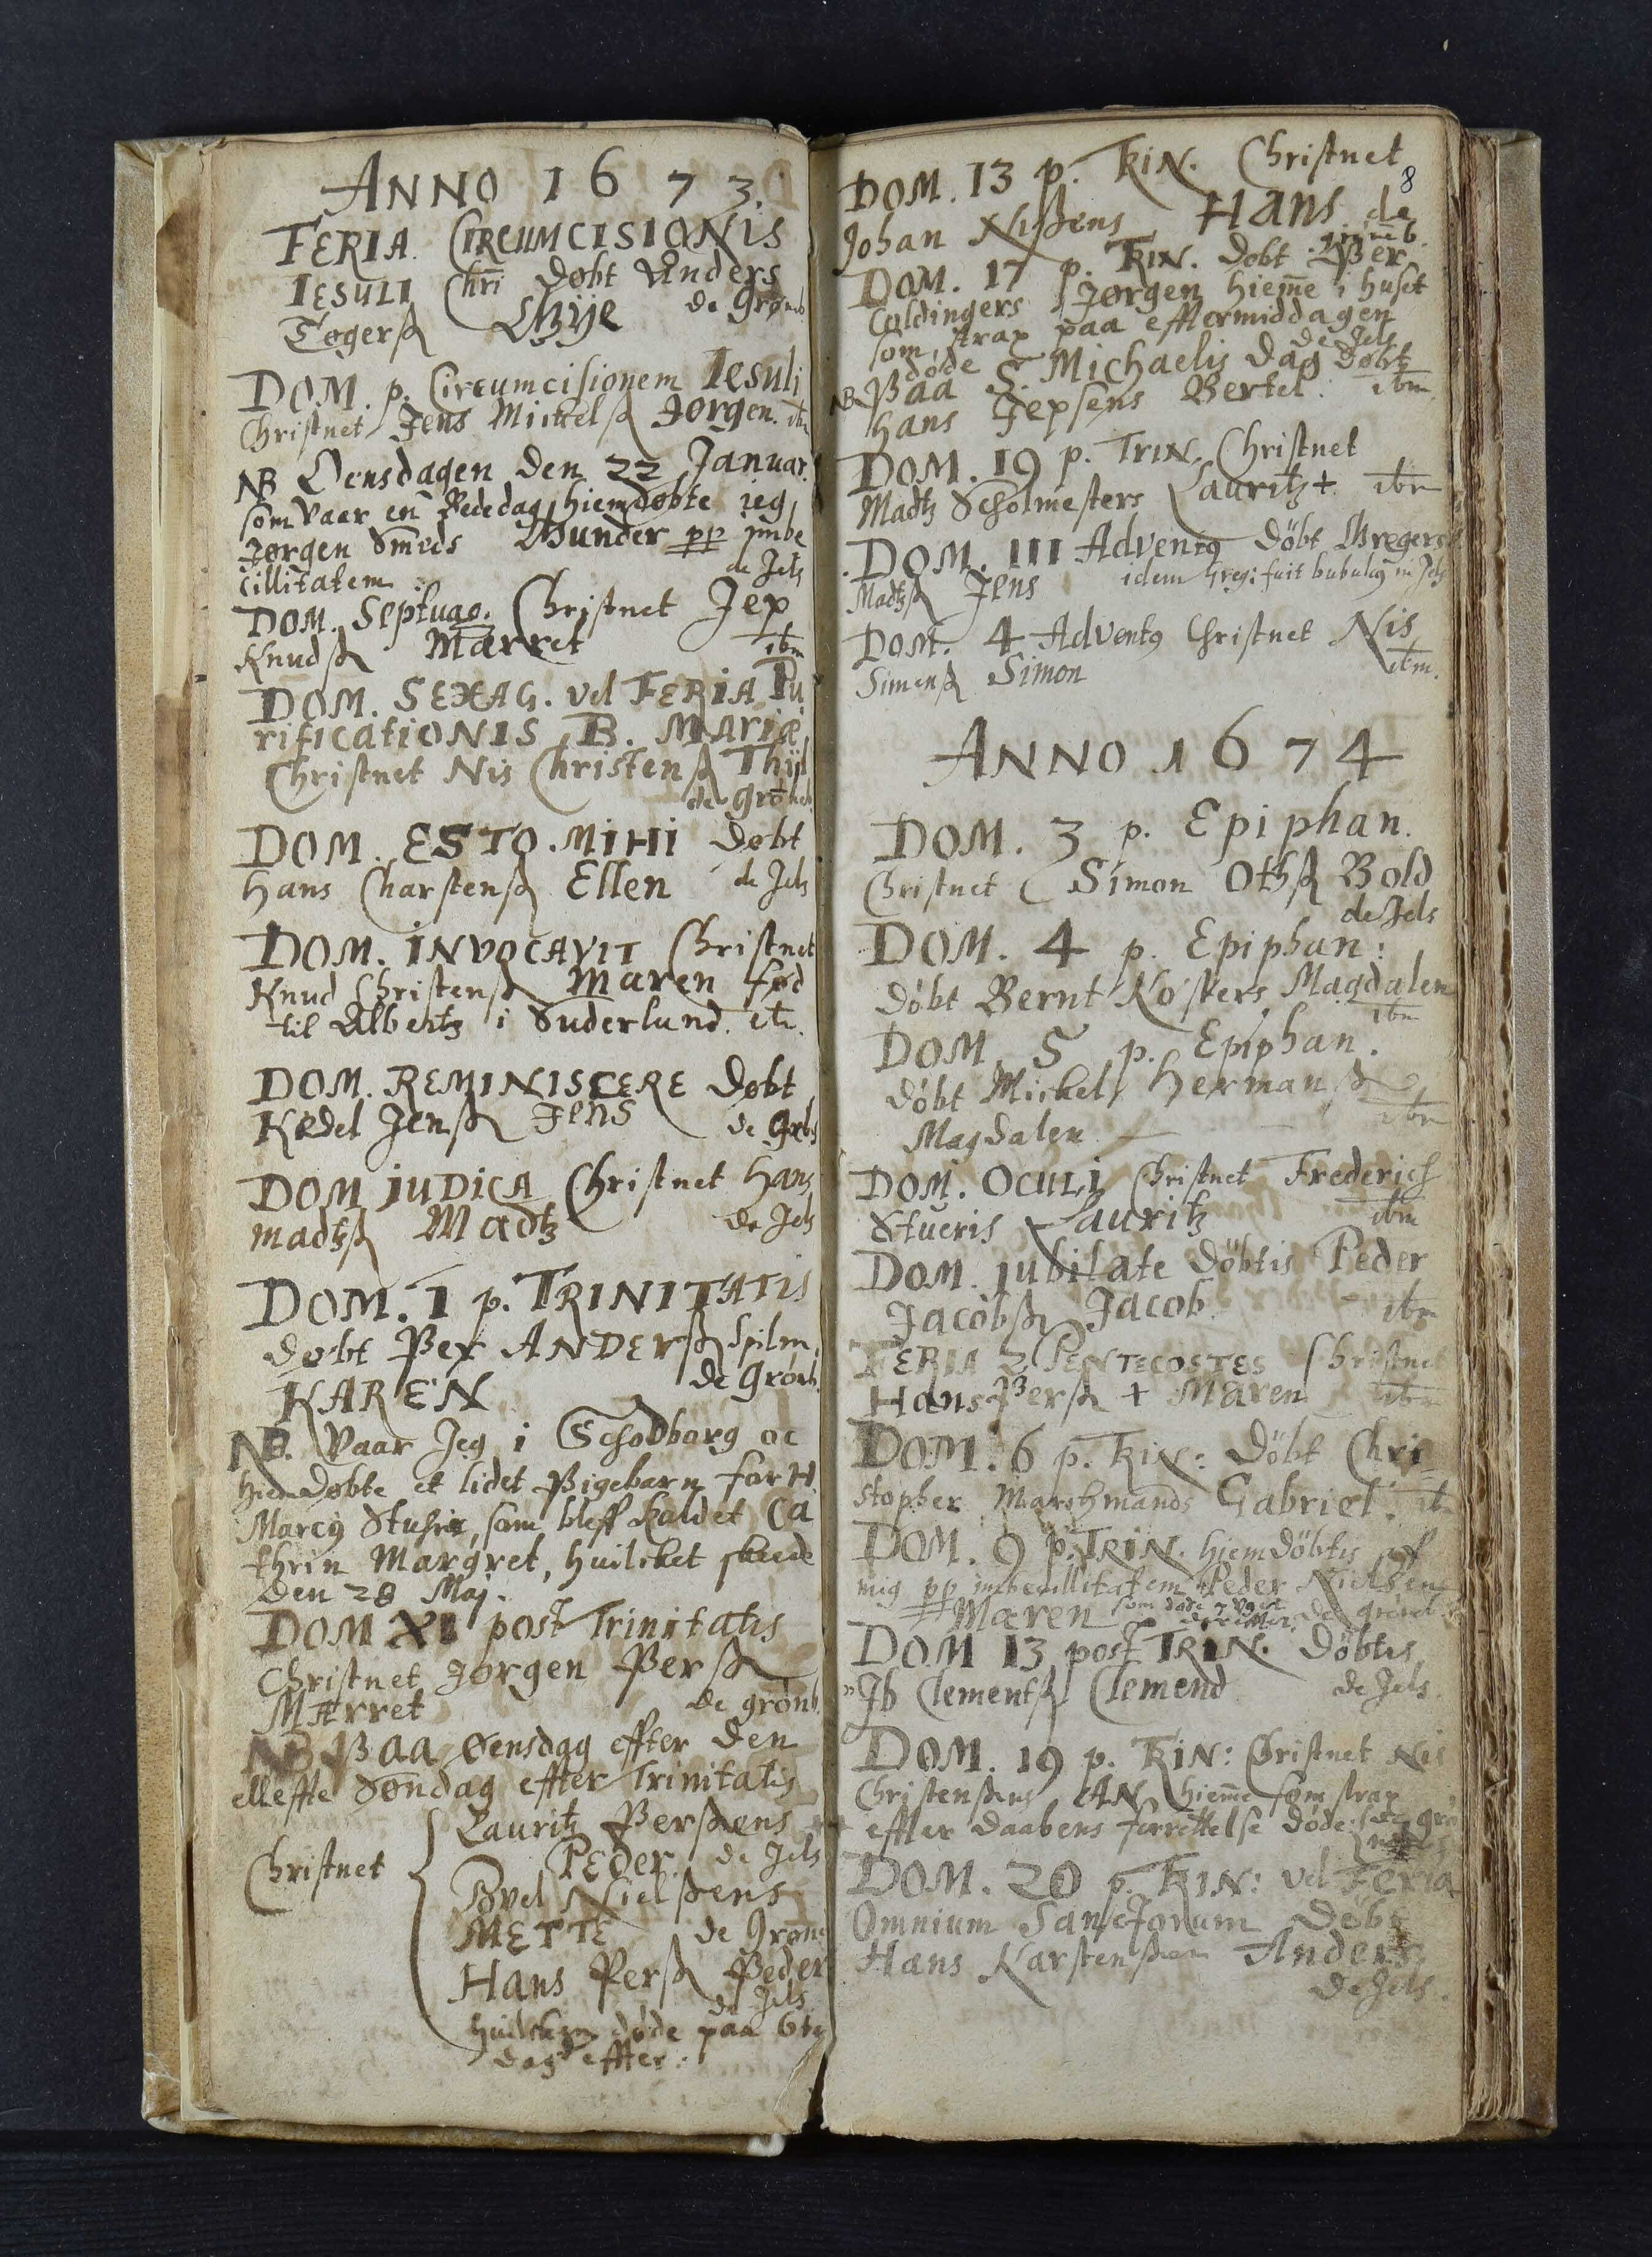ANNO 1673.
FERIA CIRCUMCISIONis
IESULI Chri døbt Anders
Thøgers Gye de Grønb
DOM. p. Circumcisionem Iesuli
Christnet Jens Mickels Jørgen ibm
NB Oensdagen den 22 Januar
som vaar en Bededag, Hiemdøbte jeg
Jørgen Smeds Gunder pp imbe
cillitatem
de Jels
DOM. Septuag. Christnet Jep
Knuds Mærret
ibm
DOM. SEXAG. ## FERIAa Pu
rificatioNIS ## Mariæ.
Christnet Nis Christens Thiil
de Grønb.
DOM. ESTO MIHI Døbt
Hans Charstens Ellen de Jels
DOM. INVOCAVIT Christnet
Knud Christens Maren fød
til Alberts i Suderlund. etc.
DOM. REMINISCERE Døbt
Kedel Jens Jens
de Grb##
DOM JUDICA Christnet Hans
Madtzs Madtz
de Jels
DOM. I p. TRINITATis
Døbt Per Anders Spilm.
KAREN
de Grønb.
NB. Vaar Jeg i Schodborg oc
Hiemdøbte et lidet pigebarn for H.
Marcq Stuhris, som bleff kaldet Ca
thrin Margret, huilket skeede
den 28 Maj.
DOM XI post Trinitatis
Christnet Jørgen Pers
Mærret
de Grønb.
NB Paa Øensdag efter den
elleffte Søndag effter Trinitatis
Christnet
Lauritz Persens
Peder de Jels
Povel Nielsens
METTE
de Grøn
Hans Pers Peder
de Jels
huilken døde paa 6te
dag effter
8
DOM. 13 p. TRIN. Christnet
Johan Nissens Hans de
Grøneb.
DOM. 17 p. TRIN. Døbt Per
Coldingers Jørgen Hieme i Huset
som strax paa eftermiddagen
døde
de Jels
NB Paa S. Michaelis Dag døbt
Hans Jepsens Bertel. Ibm.
DOM. 19 p. TRIN. Christnet
Madtz Scholmesters Lauritz + ibm
DOM. III Adventq Døbt Gregers
Madtzs Jens idem Greg: fuit bubulig## in Jels
DOM. 4 Adventq Christnet Nis
Simons Simon
ibm.
ANNO 1674
DOM. 3 p. Epiphan.
Christnet Simon Oths Bold
de Jels
DOM. 4 p. Epiphan:
Døbt Bernt Kosters Magdalen
ibm
DOM. 5 p. Epiphan.
Døbt Mickel Hermans
Magdalen
ibm
DOM. OCULI Chrisnet Frederich
Stueris Lauritz
ibm
DOM. Jubilate Døbtis Peder
Jacobs Jacob
ibm.
FERIA 2 PENTECOSTES Christnet
Hans Pers † Maren ibm
DOM. 6 p. TRIN: Døbt Chri¬
stopher MarsHmands Gabriel. ib
DOM. 9 p. TRIN. Hiem Døbtis aff
mig pp imbecillitatem Peder Nielsens
Maren
som døde 7 Vger
derefter de Grøneb.
DOM 13 post TRIN. Døbtis
Ib Clements Clemend
de Jels
DOM. 19 p. TRIN: Christnet Nis
Christensens AN Hiemme som strax
eff0ter Daabens Forrettelse døde:
de Grø
n##
Dom. 20 p. TRIN. vel Feria
Omnium Sanctorum Døbt
Hans Karstensen Anders
de Jels.
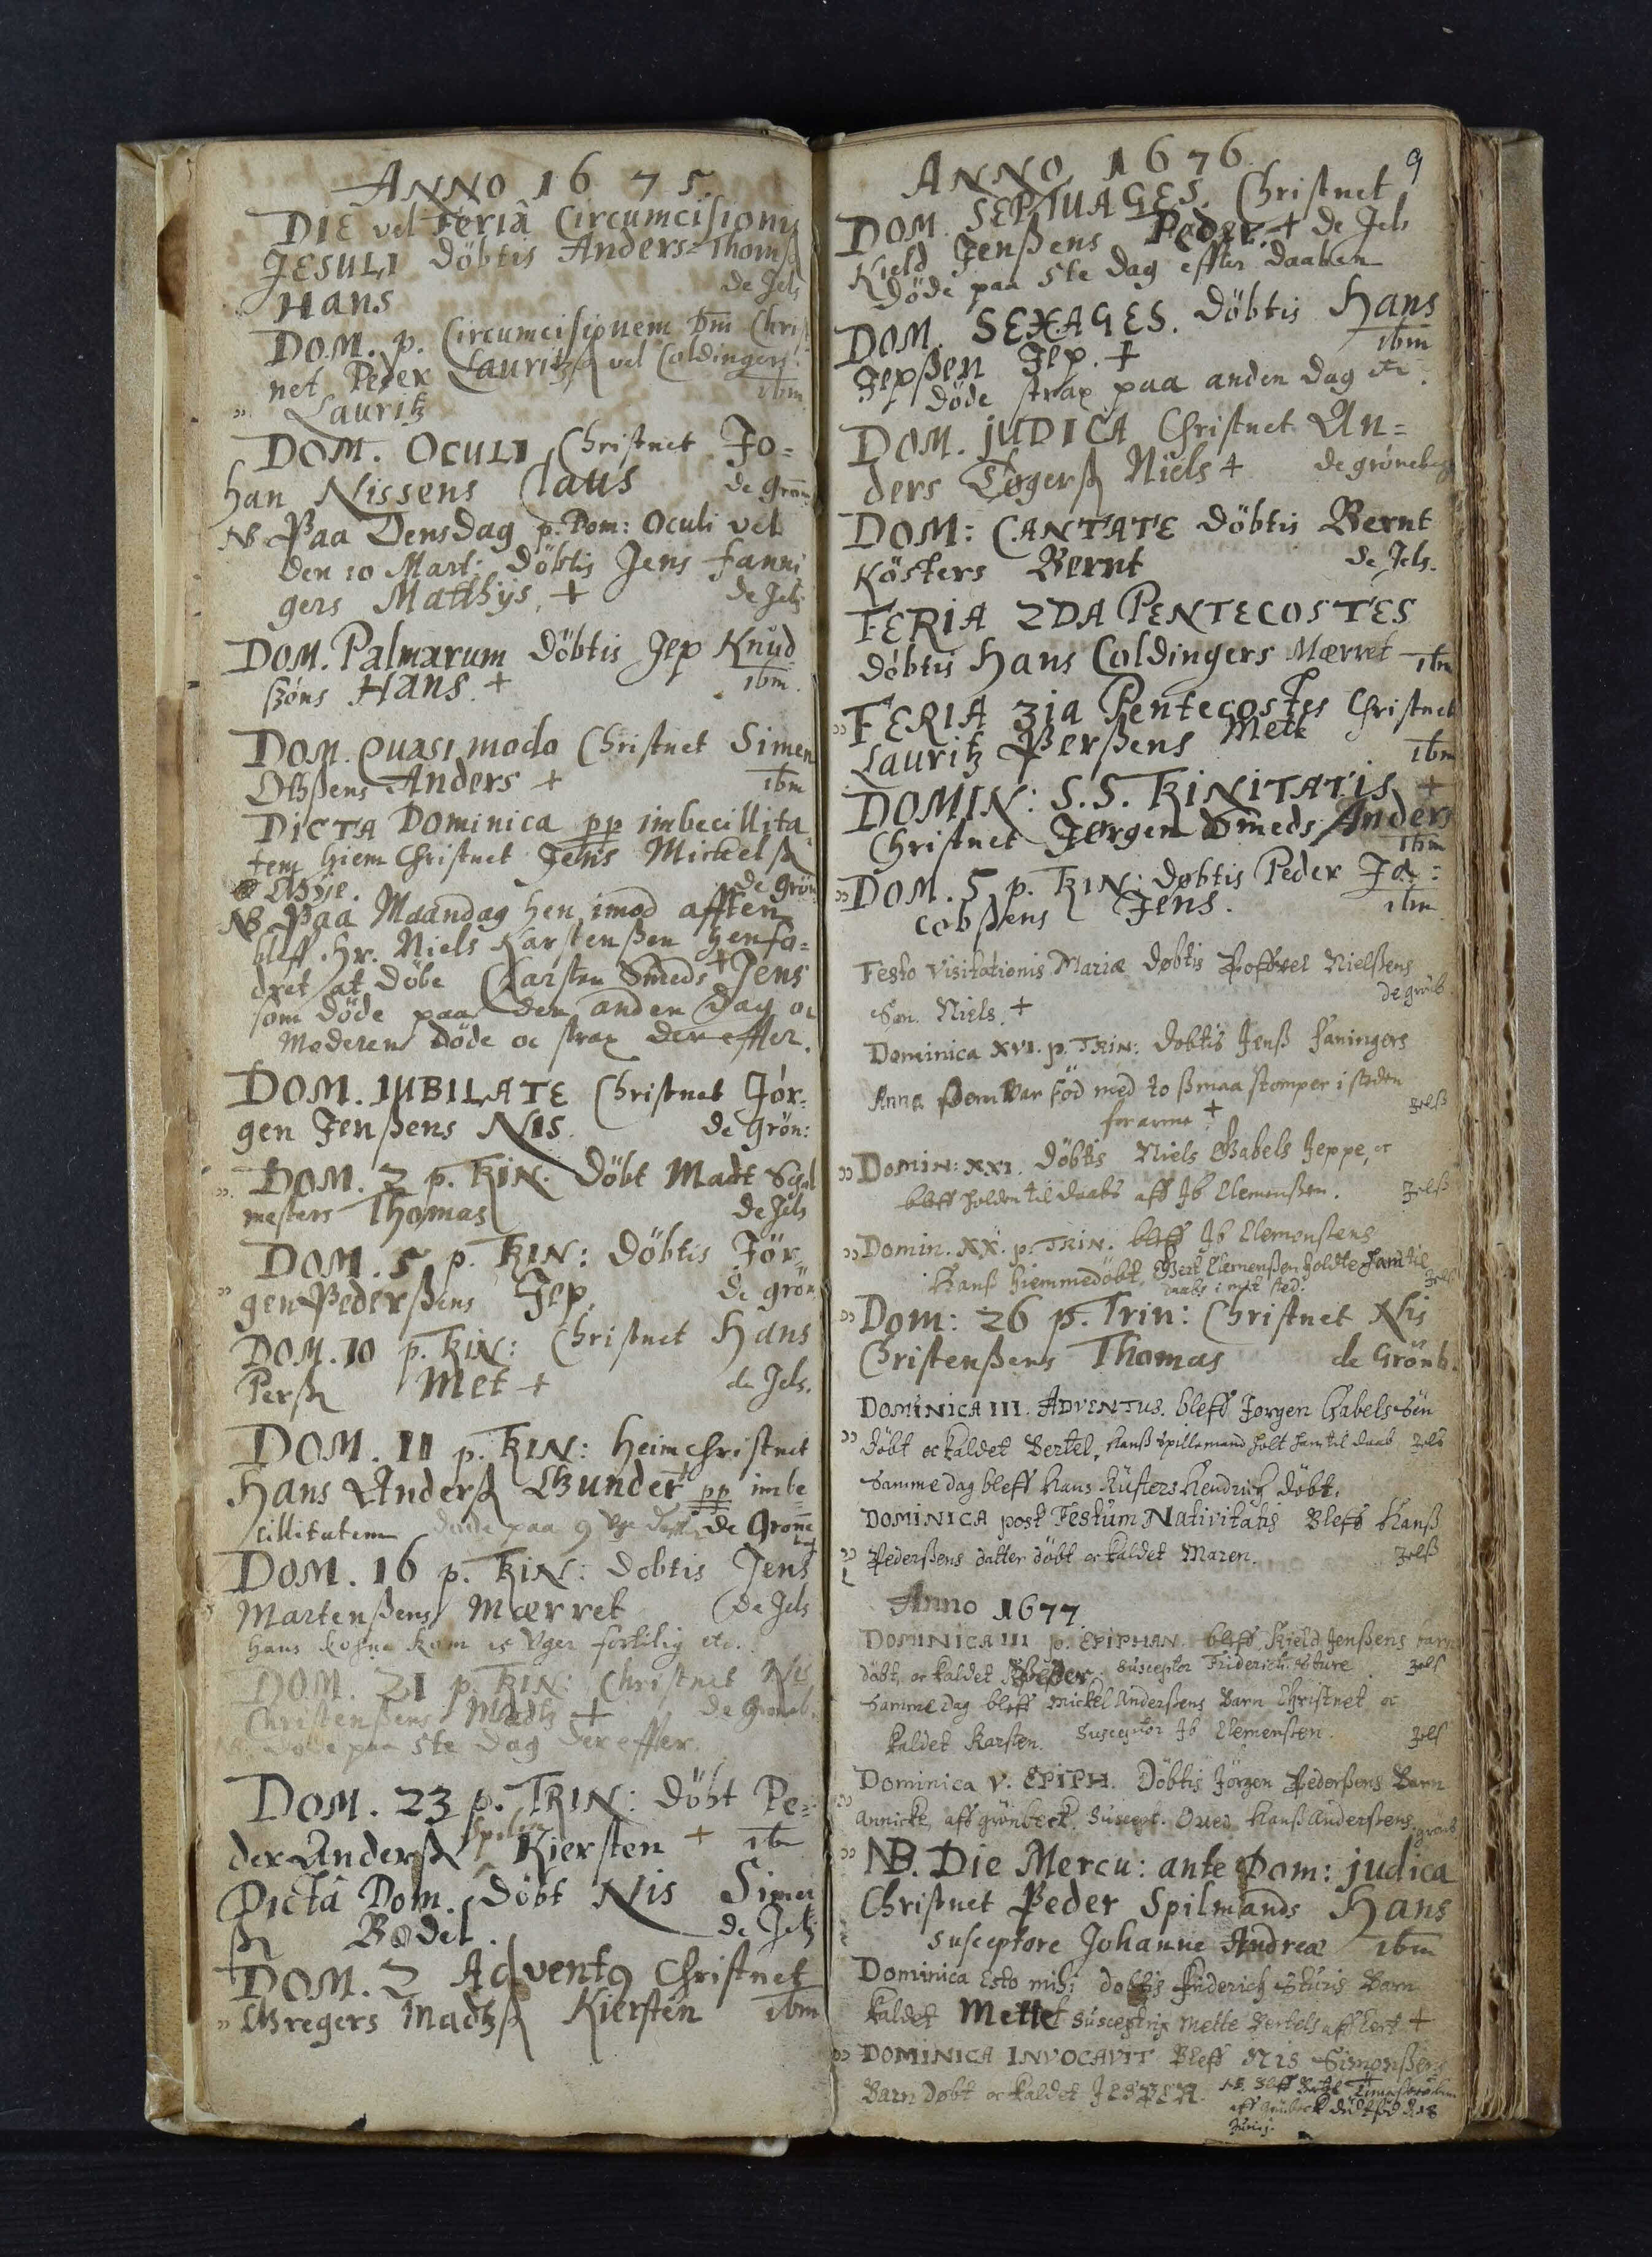ANNO 1675.
DIE vel Feria Circumcisiom
JESULI Døbtis Anders Thoms
Hans
de Jels
Do.m. p. Circumsionem Dm Christ
net Peder Lauritzs vel Coldingers
Lauritz
ibm
Dom. Oculi Christnet Jo¬
han Nissens Claus de Grøn
NB Paa Oensdag p. Dom: Oculi vel
den 10 Mart: Døbtis Jens Fanni
gers Matthijs
de Jels.
DOM. Palmarum Døbtis Jep Knud
szøns Hans
ibm
DOM. Quasimodo Christnet Simen
Othsens Anders
ibm
DICTA Dominica pp imbecillita¬
tem Hiem Christnet Jens Mickels
Gye.
de Grøn
NB Paa Maandag hen imod Afften
bleff hr. Niels Karstensen henfo¬
dret at Døbe Charsten Smeds Jens
som Døde paa den anden Dag oc
Moderen døde oc strax der effter.
DOM. JUBILATE Christnet Jør¬
gen Jensens NIS
de Grøn:
DOM. 2 p. TRIN. Døbt Madt Schol
mesters Thomas
de Jels
DOM. 5 p. TRIN: Døbtis Jør¬
gen Pedersens Jep
de Grøn
DOM. 10 p. TRIN: Christnet Hans
Pers Met
de Jels.
Dom. 11 p. TRIN: Hiemchristnet
Hans Anders Gunder pp imbe¬
cillitatem dode paa 9 Vge derefter de Grønne
bech
DOM. 16 p. TRIN: Døbtis Jens
Martensens Mærret
de Jels
Hans kohne kom 15 Vger fortilig etc.
DOM. 21 p. TRIN: Christnet Nis
Christensens Madtz
de Grøneb.
NB. Døde paa 5te Dag der effter.
DOM. 23 p. TRIN: Døbt Pe¬
Spilm
der Anders Kiersten
ibm
Dicta Dom. Døbt Nis Simon
s Bodil.
de Jels
DOM. 2 Adventq Christnet
Gregers Madtzs Kiersten ibm
9
ANNO 1676.
DOM. SEPTUAGES. Christnet
Kield Jensens Peder † de Jels
Døde paa 5te Dag effter Daaben
DOM. SEXAGES. Døbtis Hans
Jepsen Jep
ibm
Døde strax paa anden dag etc.
DOM. JUDICA Christnet An¬
ders Tøgers Niels
de grønebech
DOM: CANTATE Døbtis Bernt
Køsters Bernt
de Jels
FERIAa 2DA PENTECOSTES
Døbtis Hans Coldingers Mærret ibm
FERIA 3ia Pentecostes Christnet
Lauritz Persens Mette
ibm
DOMIN: S. S. TRINITATIS
Christnet Jørgen Smeds Anders
ibm
DOM. 5 p. TRIN: Døbtis Peder Ja¬
cobsens Jens.
ibm.
Festo Visitationis Mariæ Døbtis Pof##vel Nielsens
Søn Niels.
de grønb.
Dominica XVI p. Trin: Døbtis Jens Faningers
Anna ## Var fød med to smaa stomper i steden
for arme
Jelss
Domin: XXI Døbtis Niels Gabels Jeppe, oc
bleff holden til daabs aff Ib Clemenssen. Jelss
Domin. XX p. Trin. bleff Ib Clemensens
Hans Hiemmedøbt, Gert Clemensen holdte ham til
daabs i mit sted.
Jels
Dom: 26 p. Trin: Christnet Nis
Christensens Thomas de Grøneb.
DOMINICA III ADVENTUS. bleff Jørgen Gabels Søn
Døbt oc Kaldet Bertel, Hans Spillemand holt ham til daab. Jels
Samme dag bleff Hans Küsters Hendrichk Døbt.
DOMINICA post Festum Nativitatis Bleff Hanss
Pedersens datter døbt oc kaldet Maren.
Jelss
Anno 1677.
DOMINICA III p. EPIPHAN. Bleff Kield Jensens barn
døbt, oc kaldet Peder, susceptor Friderich Sture. Jels
Samme Dag bleff Mickel Andersens Barn Christnet oc
kaldet Karsten. Susceptor Ib Clemensen
Jels.
Dominica V. EPIPH. Døbtis Jørgen Pedersens Barn
Annicke aff grønbeck. Suscept. Oued Hans Andersens. grønb
NB. Die Marcu: ante Dom: judica
Christnet Peder Spilmands Hans
susceptore Johanne Andrea ibm
Dominica Esto mihi døbtis Friderich Sturis barn
kaldet Mette susceptrix Mette Bertels aff Lert
DOMINICA INVOCAVIT Bleff Nis Simonssens
Barn døbt oc kaldet Jesper.
NB. Bleff Bertel Timas## b##
aff Grønbeck dødfød d 18
Junij.